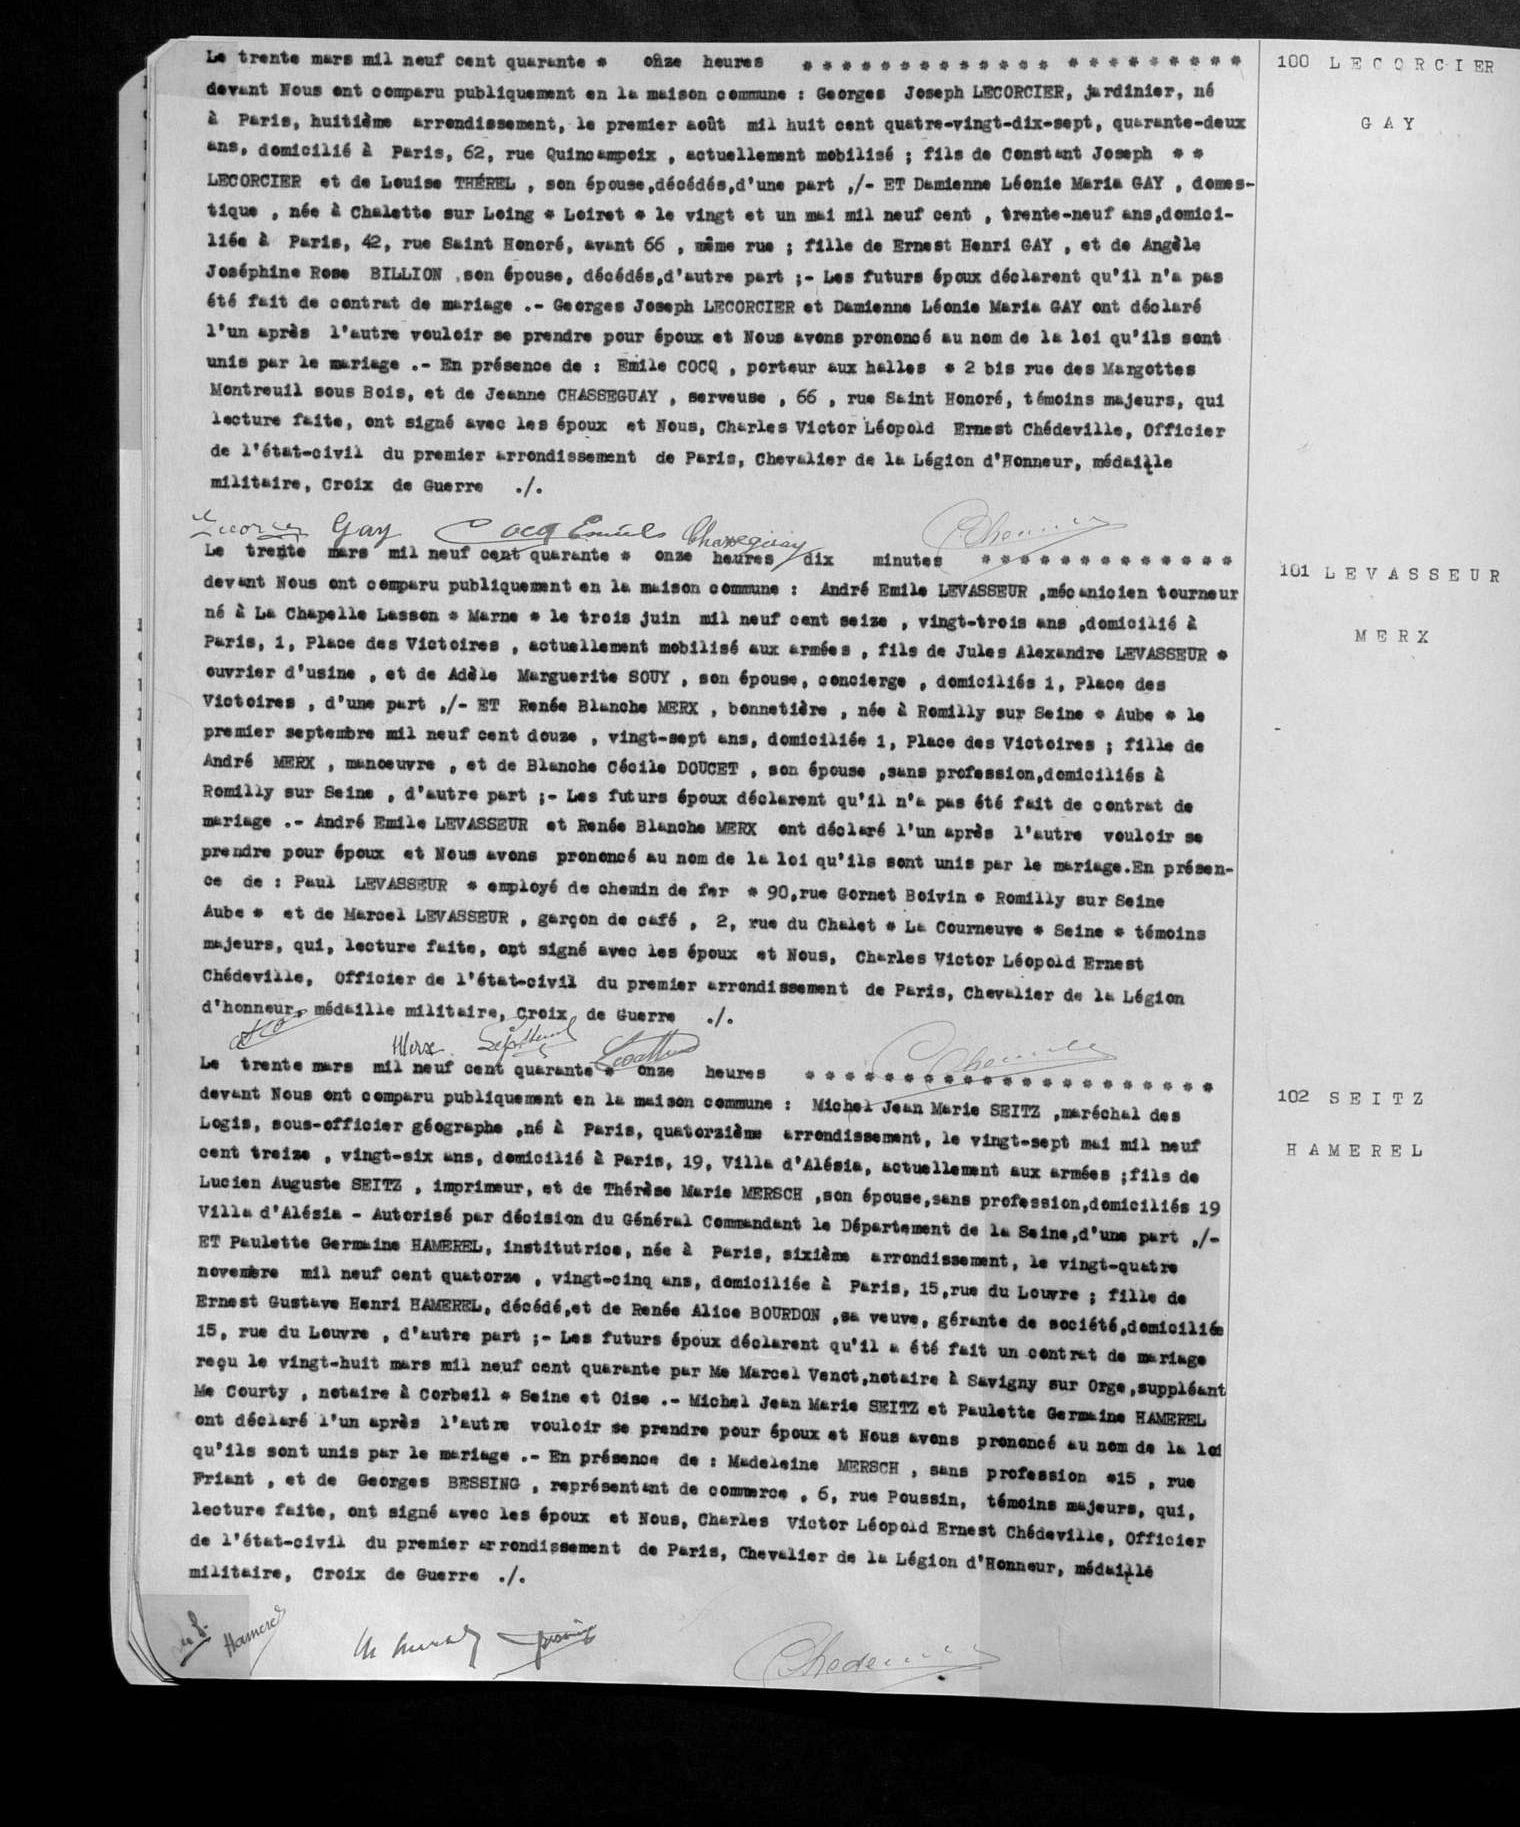Le trente mars mil neuf cent quarante * onze heures ****
devant Nous ont comparu publiquement en la maison commune: Georges Joseph LECORCIER, jardinier, né
à Paris, huitième arrondissement, le premier août mil huit cent quatre-vingt-dix-sept, quarante-deux
ans, domicilié à Paris, 62, rue Quincampoix, actuellement mobilisé; fils de Constant Joseph **
LECORCIER et de Louis THEREL, son épouse, décédés, d'une part ,/-ET Damienne Léonie Maria GAY, domes-
tique, née à Chalette sur Loing * Loiret * le vingt et un mai mil neuf cent, trente-neuf ans, domici-
liée à Paris, 42, rue Saint Honoré, avant 66, même rue; fille de Ernest Henri GAY, et de Angèle
Joséphine Rose BILLION, son épouse, décédés, d'autre part ;-Les futurs époux déclarent qu'il n'a pas
été fait de contrat de mariage .-Georges Joseph LECORCIER et Damienne Léonie Maria GAY ont déclaré
l'un après l'autre vouloir se prendre pour époux et Nous avons prononcé au nom de la loi qu'ils sont
unis par le mariage .-En présence de: Emile COCQ, porteur aux halles * 2 bis rue des Margottes
Montreuil sous Bois, et de Jean CHASSEGUAY, serveuse, 66, rue Saint Honoré, témoins majeurs, qui
lecture faite, ont signé avec les époux et Nous, Charles Victor Léopold Ernest Chédeville, Officier
de l'état-civil du premier arrondissement de Paris, Chevalier de la Légion d'Honneur, médaille
militaire, Croix de Guerre ./.
Le trente mars mil neuf cent quarante * onze heures dix minutes ****
devant Nous ont comparu publiquement en la maison commune: André Emile LEVASSEUR, mécanicien tourneur
né à La Chapelle Lasson * Marne * le trois juin mil neuf cent seize, vingt-trois ans, domicilié à
Paris, 1, Place des Victoires, actuellement mobilisé aux armées, fils de Jules Alexandre LEVASSEUR *
ouvrier d'usine, et de Adèle Marguerite SOUY, son épouse, concierge, domiciliés 1, Place des
Victoires, d'une part ,/-ET Renée Blanche MERX, bonnetière, née à Romilly sur Seine * Aube * le
premier septembre mil neuf cent douze, vingt-sept ans, domiciliée 1, Place des Victoires; fille de
André MERX, manoeuvre, et de Blanche Cécile DOUCET, son épouse, sans profession, domiciliés à
Romilly sur Seine, d'autre part ;-Les futurs époux déclarent qu'il n'a pas été fait de contrat de
mariage .-André Emile LEVASSEUR et Renée Blanche MERX ont déclaré l'un après l'autre vouloir se
prendre pour époux et Nous avons prononcé au nom de la loi qu'ils sont unis par le mariage. En présen-
ce de: Paul LEVASSEUR * employé de chemin de fer * 90, rue Gornet Boivin * Romilly sur Seine
Aube * et de Marcel LEVASSEUR, garçon de café, 2, rue du Chalet * la Courneuve * Seine * témoins
majeurs, qui, lecture faite, ont signé avec les époux et Nous, Charles Victor Léopold Ernest
Chédeville, Officier de l'état-civil du premier arrondissement de Paris, Chevalier de la Légion
d'honneur, médaille militaire, Croix de Guerre ./.
Le trente mars mil neuf cent quarante * onze heures ****
devant Nous ont comparu publiquement en la maison commune: Michel Jean Marie SEITZ, maréchal des
Logis, sous-officier géographe, né à Paris, quatorzième arrondissement, le vingt-sept mai mil neuf
cent treize, vingt-six ans, domicilié à Paris, 19, Villa d'Alésia, actuellement aux armées; fils de
Lucien Auguste SEITZ, imprimeur, et de Thérèse Marie MERSCH, son épouse, sans profession, domiciliés 19
Villa d'Alésia-Autorisé par décision du Général Commandant le Département de la Seine, d'une part ,/
ET Paulette Germaine HAMEREL, institutrice, né à Paris, sixième arrondissement, le vingt-quatre
novembre mil neuf cent quatorze, vingt-cinq ans, domiciliée à Paris, 15, rue du Louvre; fille de
Ernest Gustave Henri HAMEREL, décédé, et de Renée Alice BOURDON, sa veuve, gérante de société, domiciliée
15, rue du Louvre, d'autre part ;-Les futurs époux déclarent qu'il a été fait un contrat de mariage
reçu le vingt-huit mars mil neuf cent quarante par Me Marcel Venot, notaire à Savigny sur Orge, suppléant
Me Courty, notaire à Corbeil * Seine et Oise .-Michel Jean Marie SEITZ et Paulette Germaine HAMEREL
ont déclaré l'un après l'autre vouloir se prendre pour époux et Nous avons prononcé au nom de la loi
qu'ils sont unis par le mariage .-En présence de: Madeleine MERSCH, sans profession * 15, rue
Friant, et de Georges BESSING, représentant de commerce, 6, rue Poussin, témoins majeurs, qui,
lecture faite, ont signé avec les époux et Nous, Charles Victor Léopold Ernest Chédeville, Officier
de l'état-civil du premier arrondissement de Paris, Chevalier de la Légion d'Honneur, médaille
militaire, Croix de Guerre ./.
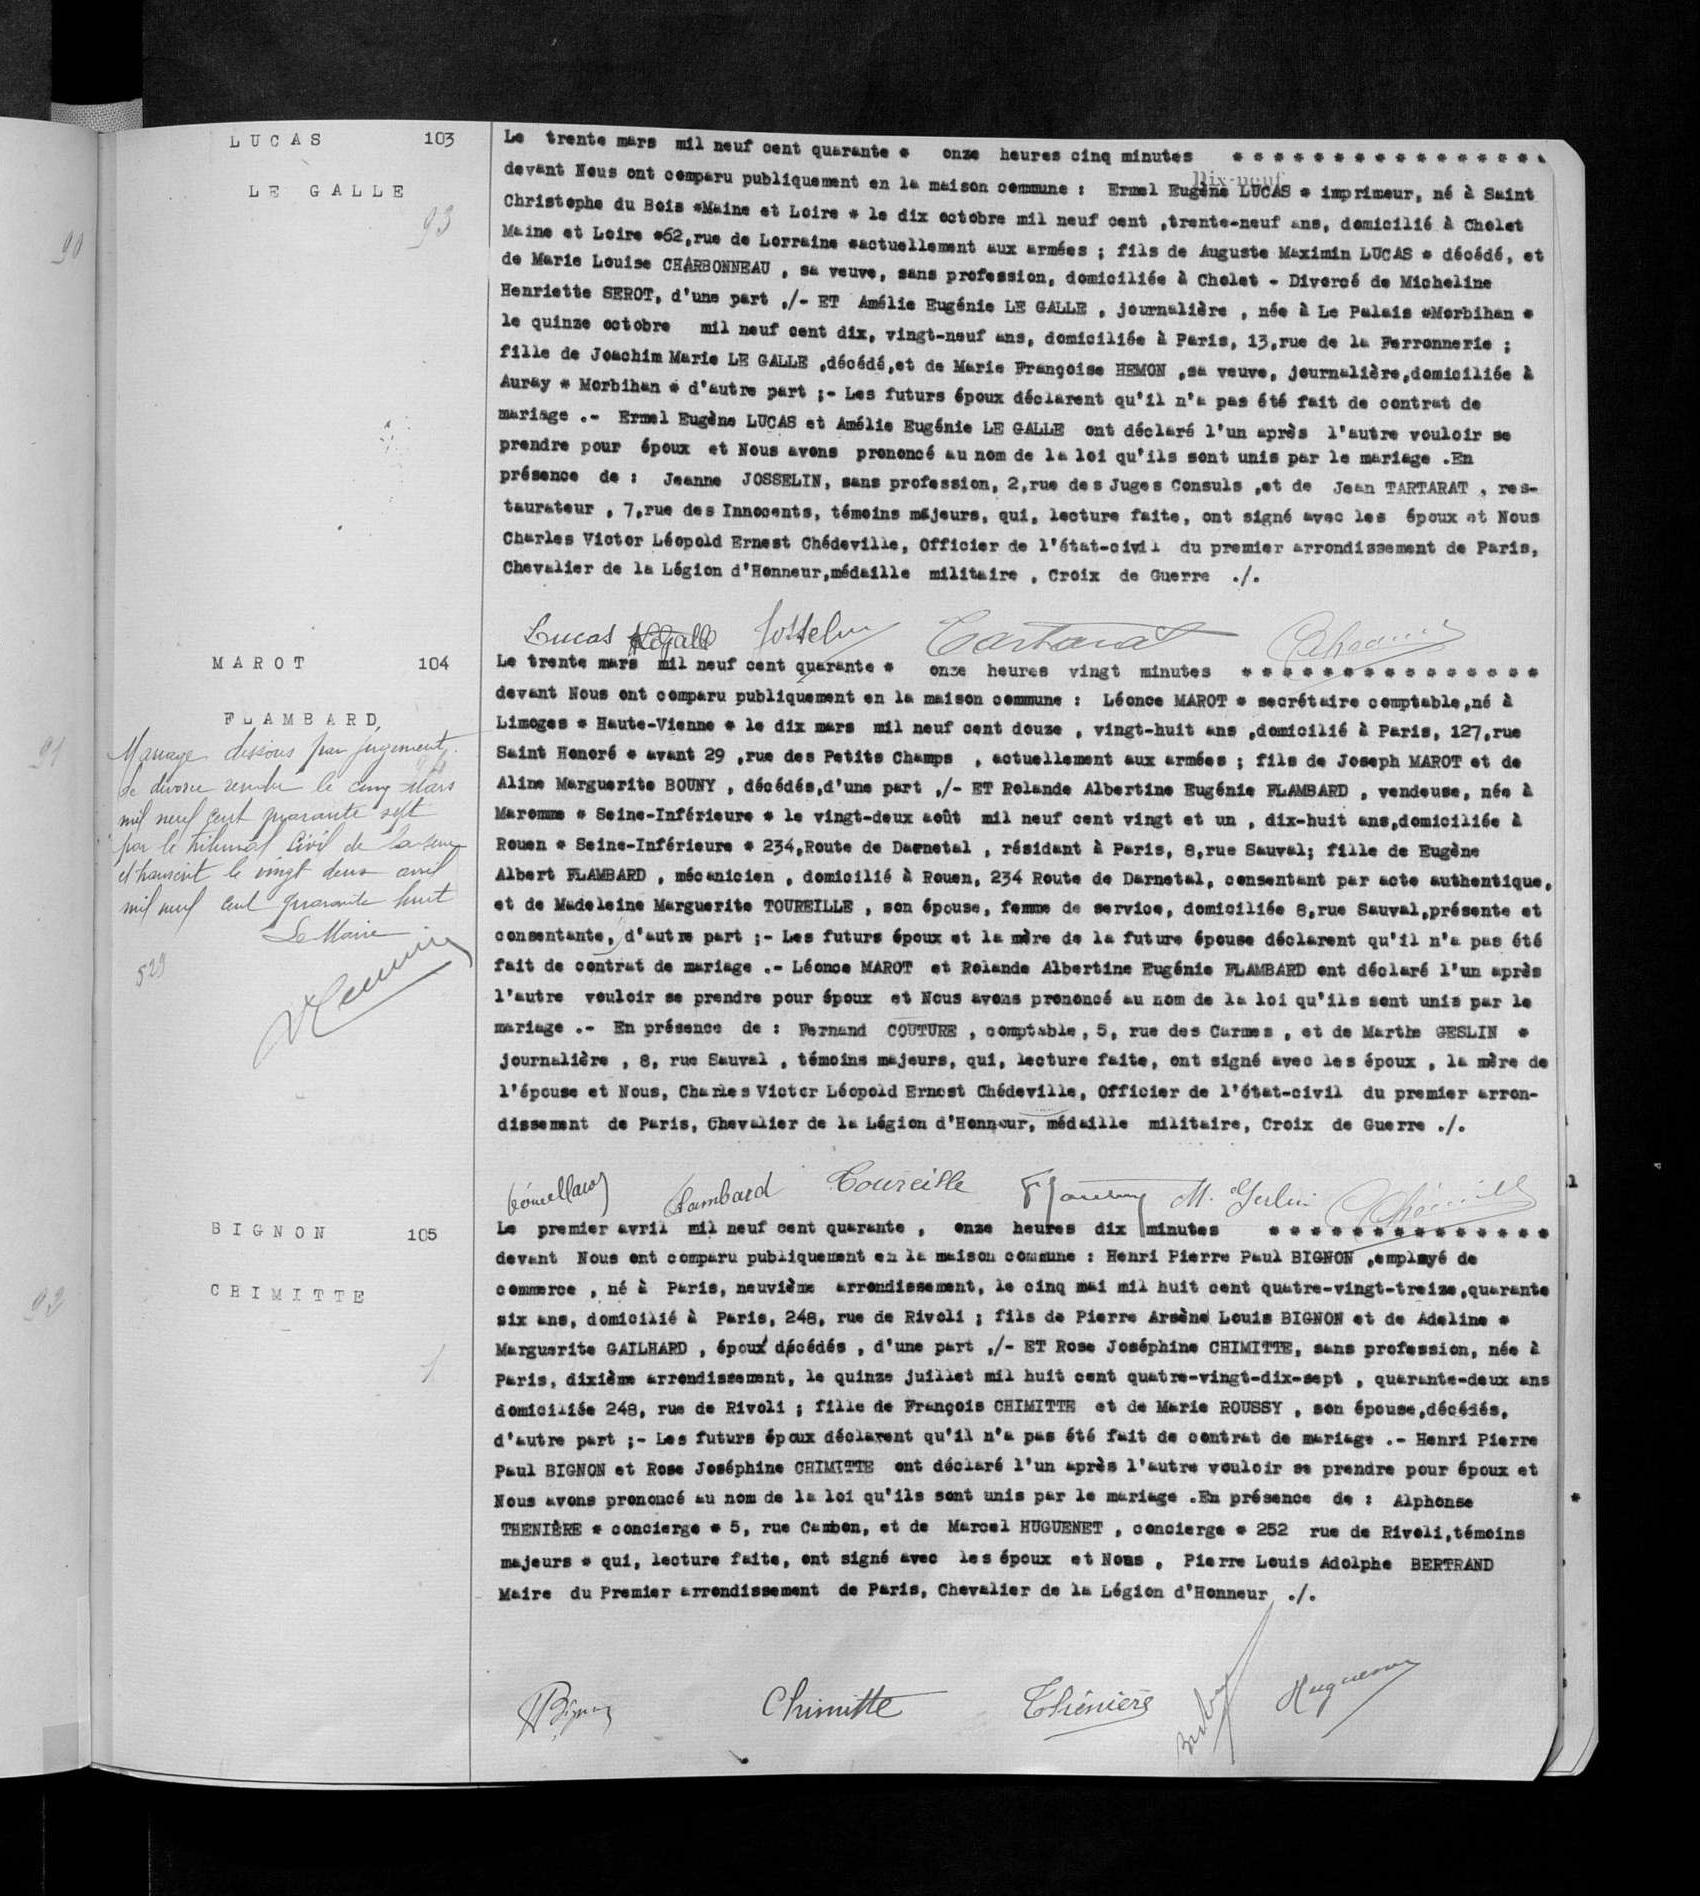Le trente mars mil neuf cent quarante * onze heures cinq minutes ****
devant Nous ont comparu publiquement en la maison commune: Ermel Eugène LUCAS * imprimeur né à Saint
Christophe du Bois * Maine et Loire * le dix octobre mil neuf cent, trente-neuf ans, domicilié à Cholet
Maine et Loir * 62, rue de Lorraine * actuellement aux armées; fils de Auguste Maximin LUCAS * décédé, et
de Marie Louise CHARBONNEAU, sa veuve, sans profession, domiciliée à Cholet Divorcé de Micheline
Henriette SEROT, d'une part ,/-ET Amélie Eugénie LE GALLE, journalière, née à Le Palais * Morbihan *
le quinze octobre mil neuf cent dix, vingt-neuf ans, domiciliée à Paris, 13, rue de la Ferronnerie;
fille de Joachim Marie LE GALLE, décédé, et de Marie Françoise HEMON, sa veuve, journalière, domiciliée à
Auray * Morbihan * d'autre part ;-Les futurs époux déclarent qu'il n'a pas été fait de contrat de
mariage .-Ermel Eugène LUCAS et Amélie Eugénie LE GALLE ont déclaré l'un après l'autre vouloir se
prendre pour époux et Nous avons prononcé au nom de la loi qu'ils sont unis par le mariage. En
présence de: Jeanne JOSSELIN, sans profession, 2, rue des Juges Consuls, et de Jean TARTARAT, res-
taurateur, 7, rue des Innocents, témoins majeurs, qui, lecture faite, ont signé avec les époux et Nous
Charles Victor Léopold Ernest Chédeville, Officier de l'état-civil du premier arrondissement de Paris,
Chevalier de la Légion d'Honneur, médaille militaire, Croix de Guerre ./.
Le trente mars mil neuf cent quarante * onze heures vingt minutes ****
devant Nous ont comparu publiquement en la maison commune: Léonce MAROT * secrétaire comptable, né à
Limoges * Haute-Vienne * le dix mars mil neuf cent douze, vingt-huit ans, domicilié à Paris, 127, rue
Saint Honoré * avant 29, rue des Petits Champs, actuellement aux armées; fils de Joseph MAROT et de
Aline Marguerite BOUNY, décdés, d'une part ,/-ET Rolande Albertine Eugénie FLAMBARD, vendeuse, née à
Maromme * Seine-Inférieure * le vingt-deux août mil neuf cent vingt et un, dix-huit ans, domiciliée à
Rouen * Seine-Inférieure * 234, Route de Darnetal, résidant à Paris, 8, rue Sauval; fille de Eugène
Albert FLAMBARD, mécanicien, domicilié à Rouen, 234 Route de Darnetal, consentant par acte authentique, et
de Madelein Marguerite TOUREILLE, son épouse, femme de service, domiciliée 8, rue Sauval, présente et
consentante, d'autre part ;-Les futurs époux et la mère de la future épouse déclarent qu'il n'a pas été
fait de contrat de mariage .-Léonce MAROT et Rolande Albertine Eugénie FLAMBARD ont déclaré l'un après
l'autre vouloir se prendre pour époux et Nous avons prononcé au nom de la loi qu'ils sont unis par le
mariage .-En présence de: Fernand COUTURE, comptable, 5, rue des Carmes, et de Marthe GESLIN *
journalière, 8, rue Sauval, témoins majeurs, qui, lecture faite, ont signé avec les époux, la mère de
l'épouse et Nous, Charles Victor Léopold Ernest Chédeville, Officier de l'état-civil du premier arron-
dissement de Paris, Chevalier de la Légion d'Honneur, médaille militaire, Croix de Guerre ./
Le premier avril mil neuf cent quarante, onze heures dix minutes ****
devant Nous ont comparu publiquement en la maison commune: Henri Pierre Paul BIGNON, employé de
commerce, né à Paris, neuvième arrondissement, le cinq mai mil huit cent quatre-vingt-treize, quarante
six ans domicilié à Paris, 248, rue de Rivoli; fils de Pierre Arsène Louis BIGNON et de Adeline *
Marguerite GAILHARD, époux décédés, d'une part ,/-ET Rose Joséphine CHIMITTE, sans profession, née à
Paris, dixième arrondissement, le quinze juillet mil huit cent quatre-vingt-dix-sept, quarante-deux ans
domiciliée 248, rue de Rivoli; fille de François CHIMITTE et de Marie ROUSSY, son épouse, décédés,
d'autre part ;-Les futurs époux déclarent qu'il n'a pas été fait de contrat de mariage .-Henri Pierre
Paul BIGNON et Rose Joséphine CHIMITTE ont déclaré l'un après l'autre vouloir se prendre pour époux et
Nous avons prononcé au nom de la loi qu'ils sont unis par le mariage. En présence de: Alphonse
THENIERE * concierge * 5, rue Cambon, et de Marcel HUGUENET, concierge * 252 rue de Rivoli, témoins
majeurs * qui, lecture faite, ont signé avec les époux et Nous, Pierre Louis Adolphe BERTRAND
Maire du Premier arrondissement de Paris, Chevalier de la Légion d'Honneur ./.